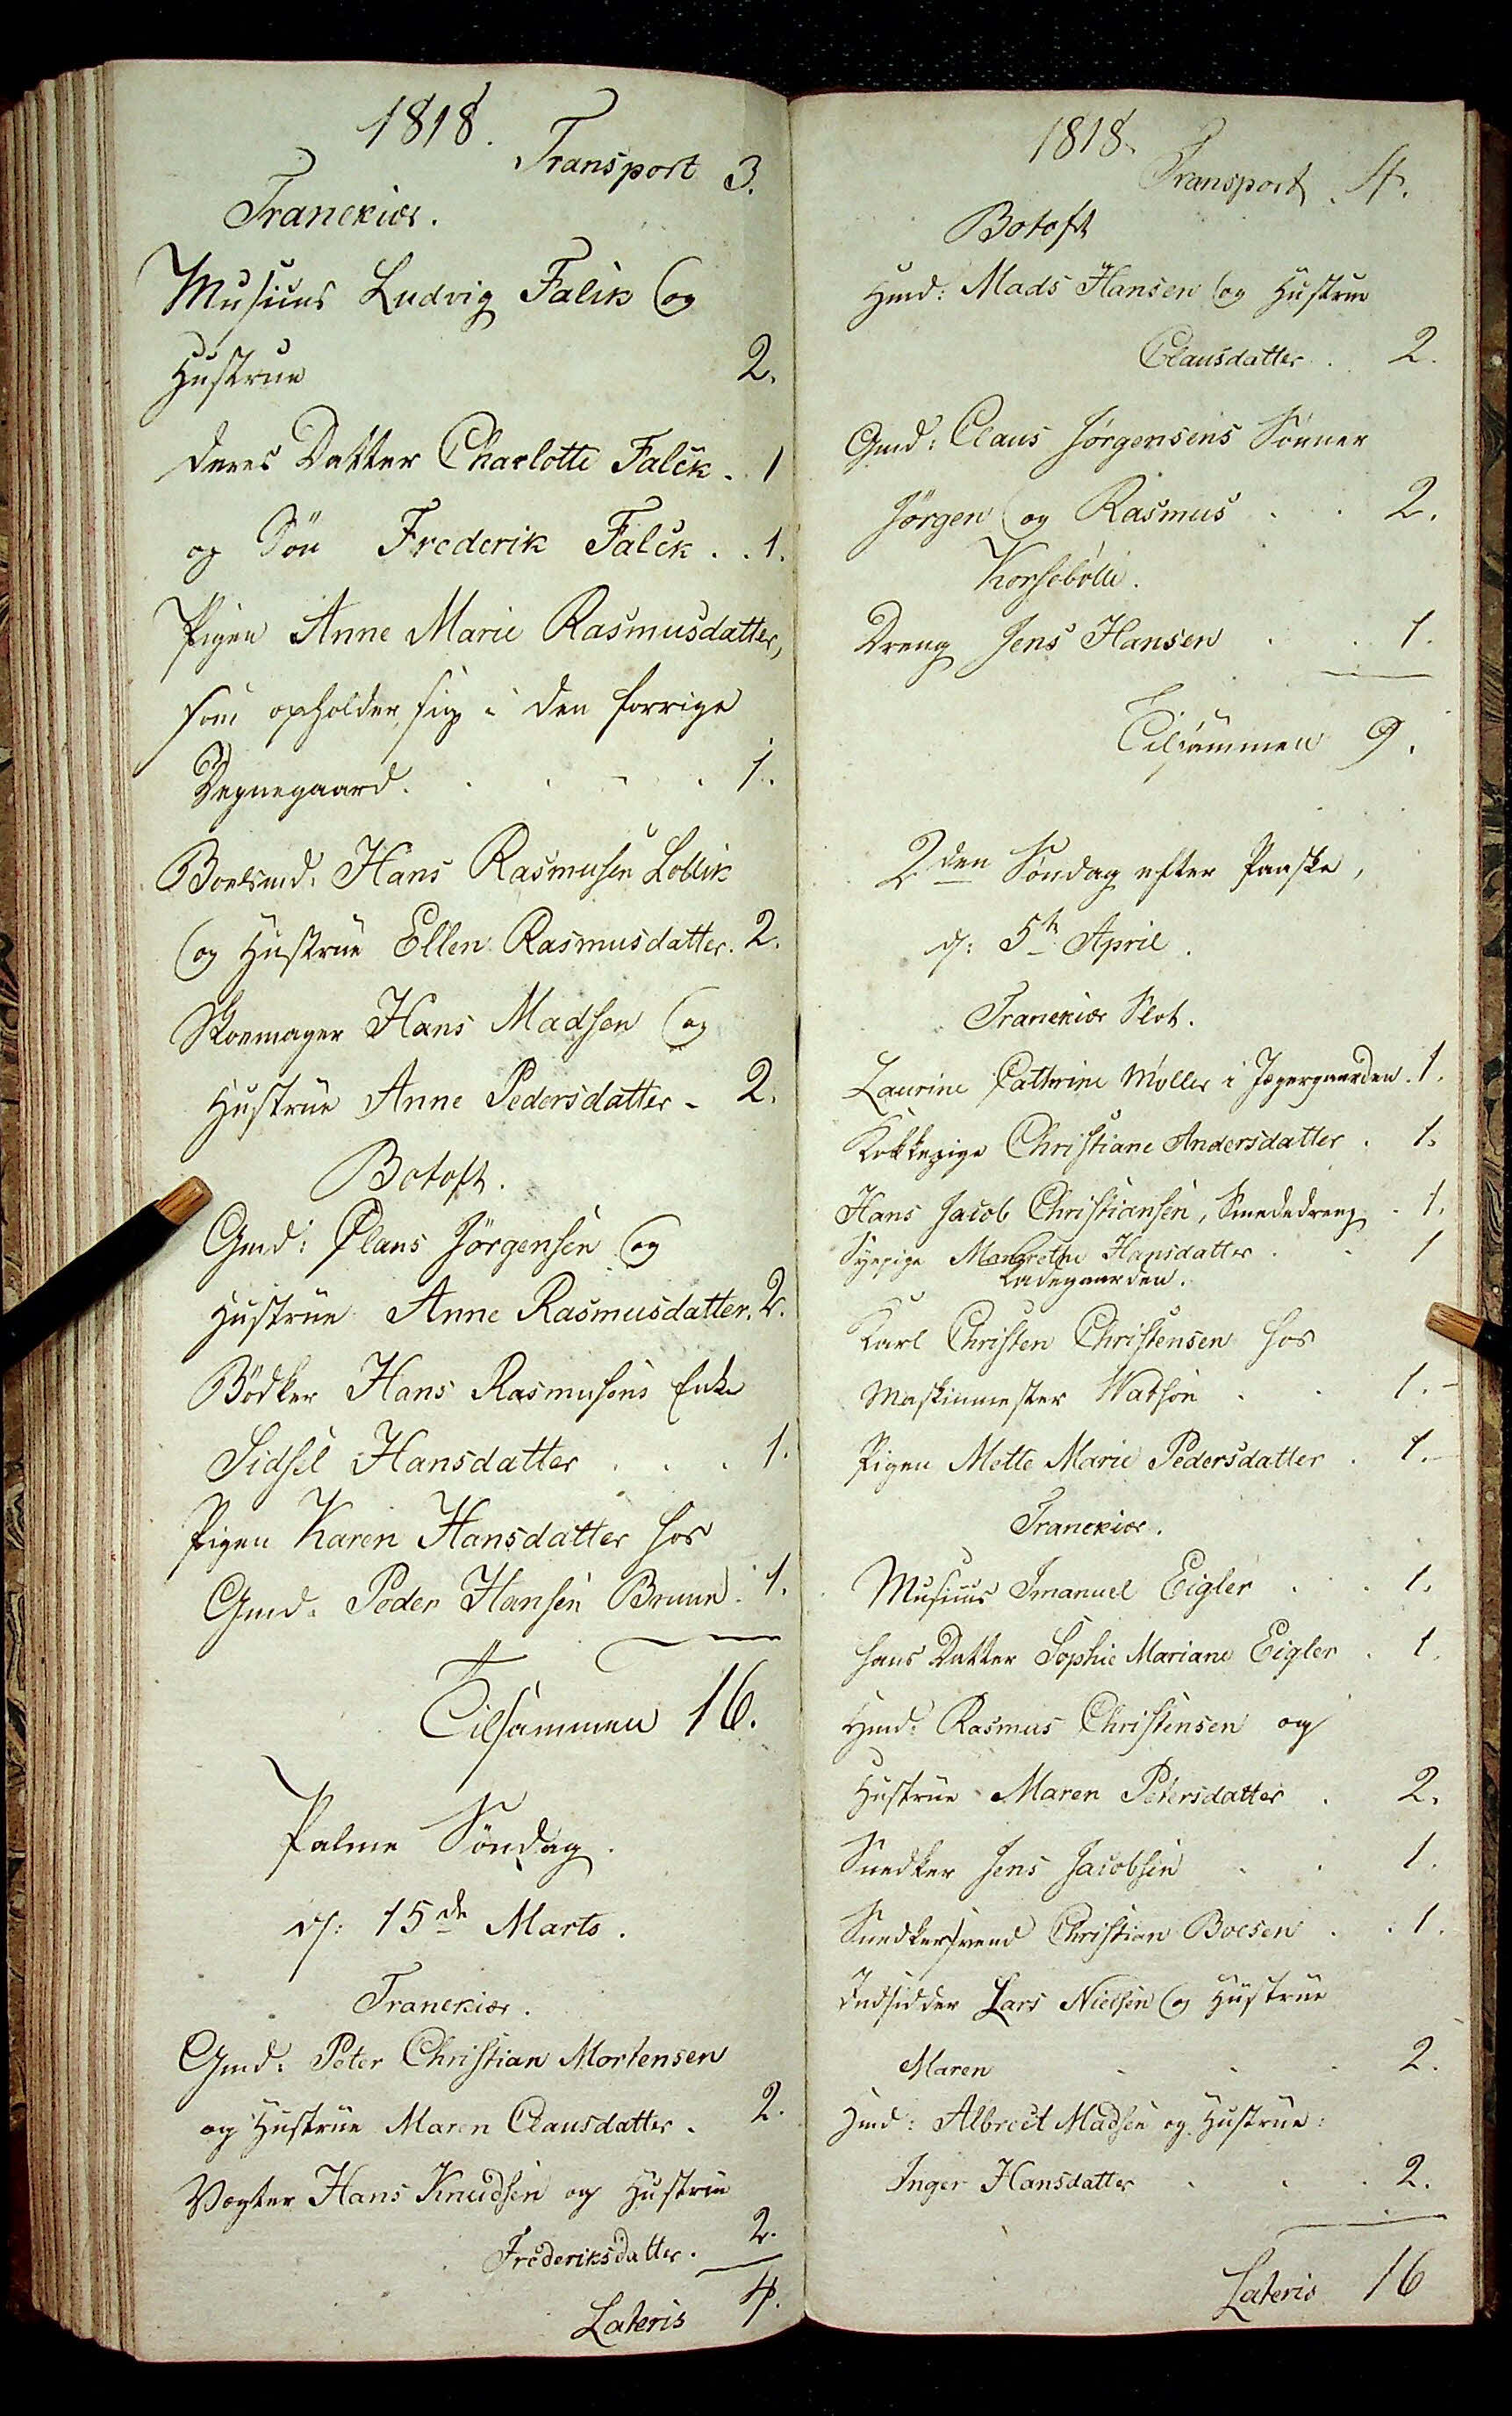1818.
Transport 3.
Tranekiær.
Musicus Ludvig Falck og
Hustrue 2.
deres Datter Charlotte Falck . 1
og Søn Friderik Falck . . 1.
Pigen Anne Marie Rasmusdatter,
som opholder sig i den forrige
Degnegaard . . . . . 1.
Boelsmd: Hans Rasmusen Lollik
og Hustrue Ellen Rasmusdatter . 2.
Skoemager Hans Madsen og
Hustrue Anne Pedersdatter - 2.
Botoft.
Gmd: Claus Jørgensen og
Hustrue Anne Rasmusdatter . 2.
Bødker Hans Rasmusens Enke
Sidsel Hansdatter . . . 1.
Pigen Karen Hansdatter hos
Gmd: Peder Hansen Bruun . 1.
Tilsammen 16.
Palme Søndag
d: 15de Marts.
Tranekiær.
Gmd: Peter Christian Mortensen
og Hustrue Maren Clausdatter . 2.
Vægter Hans Knudsen og Hustrue
Frederiksdatter . 2.
Lateris 4.
1818.
Transport . 4.
Botoft
Hmd: Mads Hansen og Hustrue
Clausdatter . 2.
Gmd: Claus Jørgensøns Sønner
Jørgen og Rasmus . . 2.
Korsebølle.
Dreng Jens Hansen . . 1.
Tilsammen 9.
2den Søndag efter Paaske,
d: 5te April
Tranekiær Slot.
Laurine Cathrine Møller i Jægergaarden . 1.
Kokkepige Christiane Andersdatter . 1.
Hans Jacob Christiansen, Smededreng . 1.
Syepige Margrethe Hansdatter . . 1
Ladegaarden.
Karl Christen Christensen hos
Maskinmester Watson . . 1.
Pigen Mette Marie Pedersdatter . 1.
Tranekiær.
Musicus Imanuel Eigler . . 1.
hans Datter Sophie Mariane Eigler . 1.
Hmd: Rasmus Christensen og
Hustrue Maren Petersdatter . 2.
Snedker Jens Jacobsen . . 1
Snedkersvend Christian Boesen . . 1
Indsidder Lars Nielsen og Hustrue
Maren . . . 2.
Hmd: Albrect Madsen og Hustrue:
Inger Hansdatter . . . 2.
Lateris 16
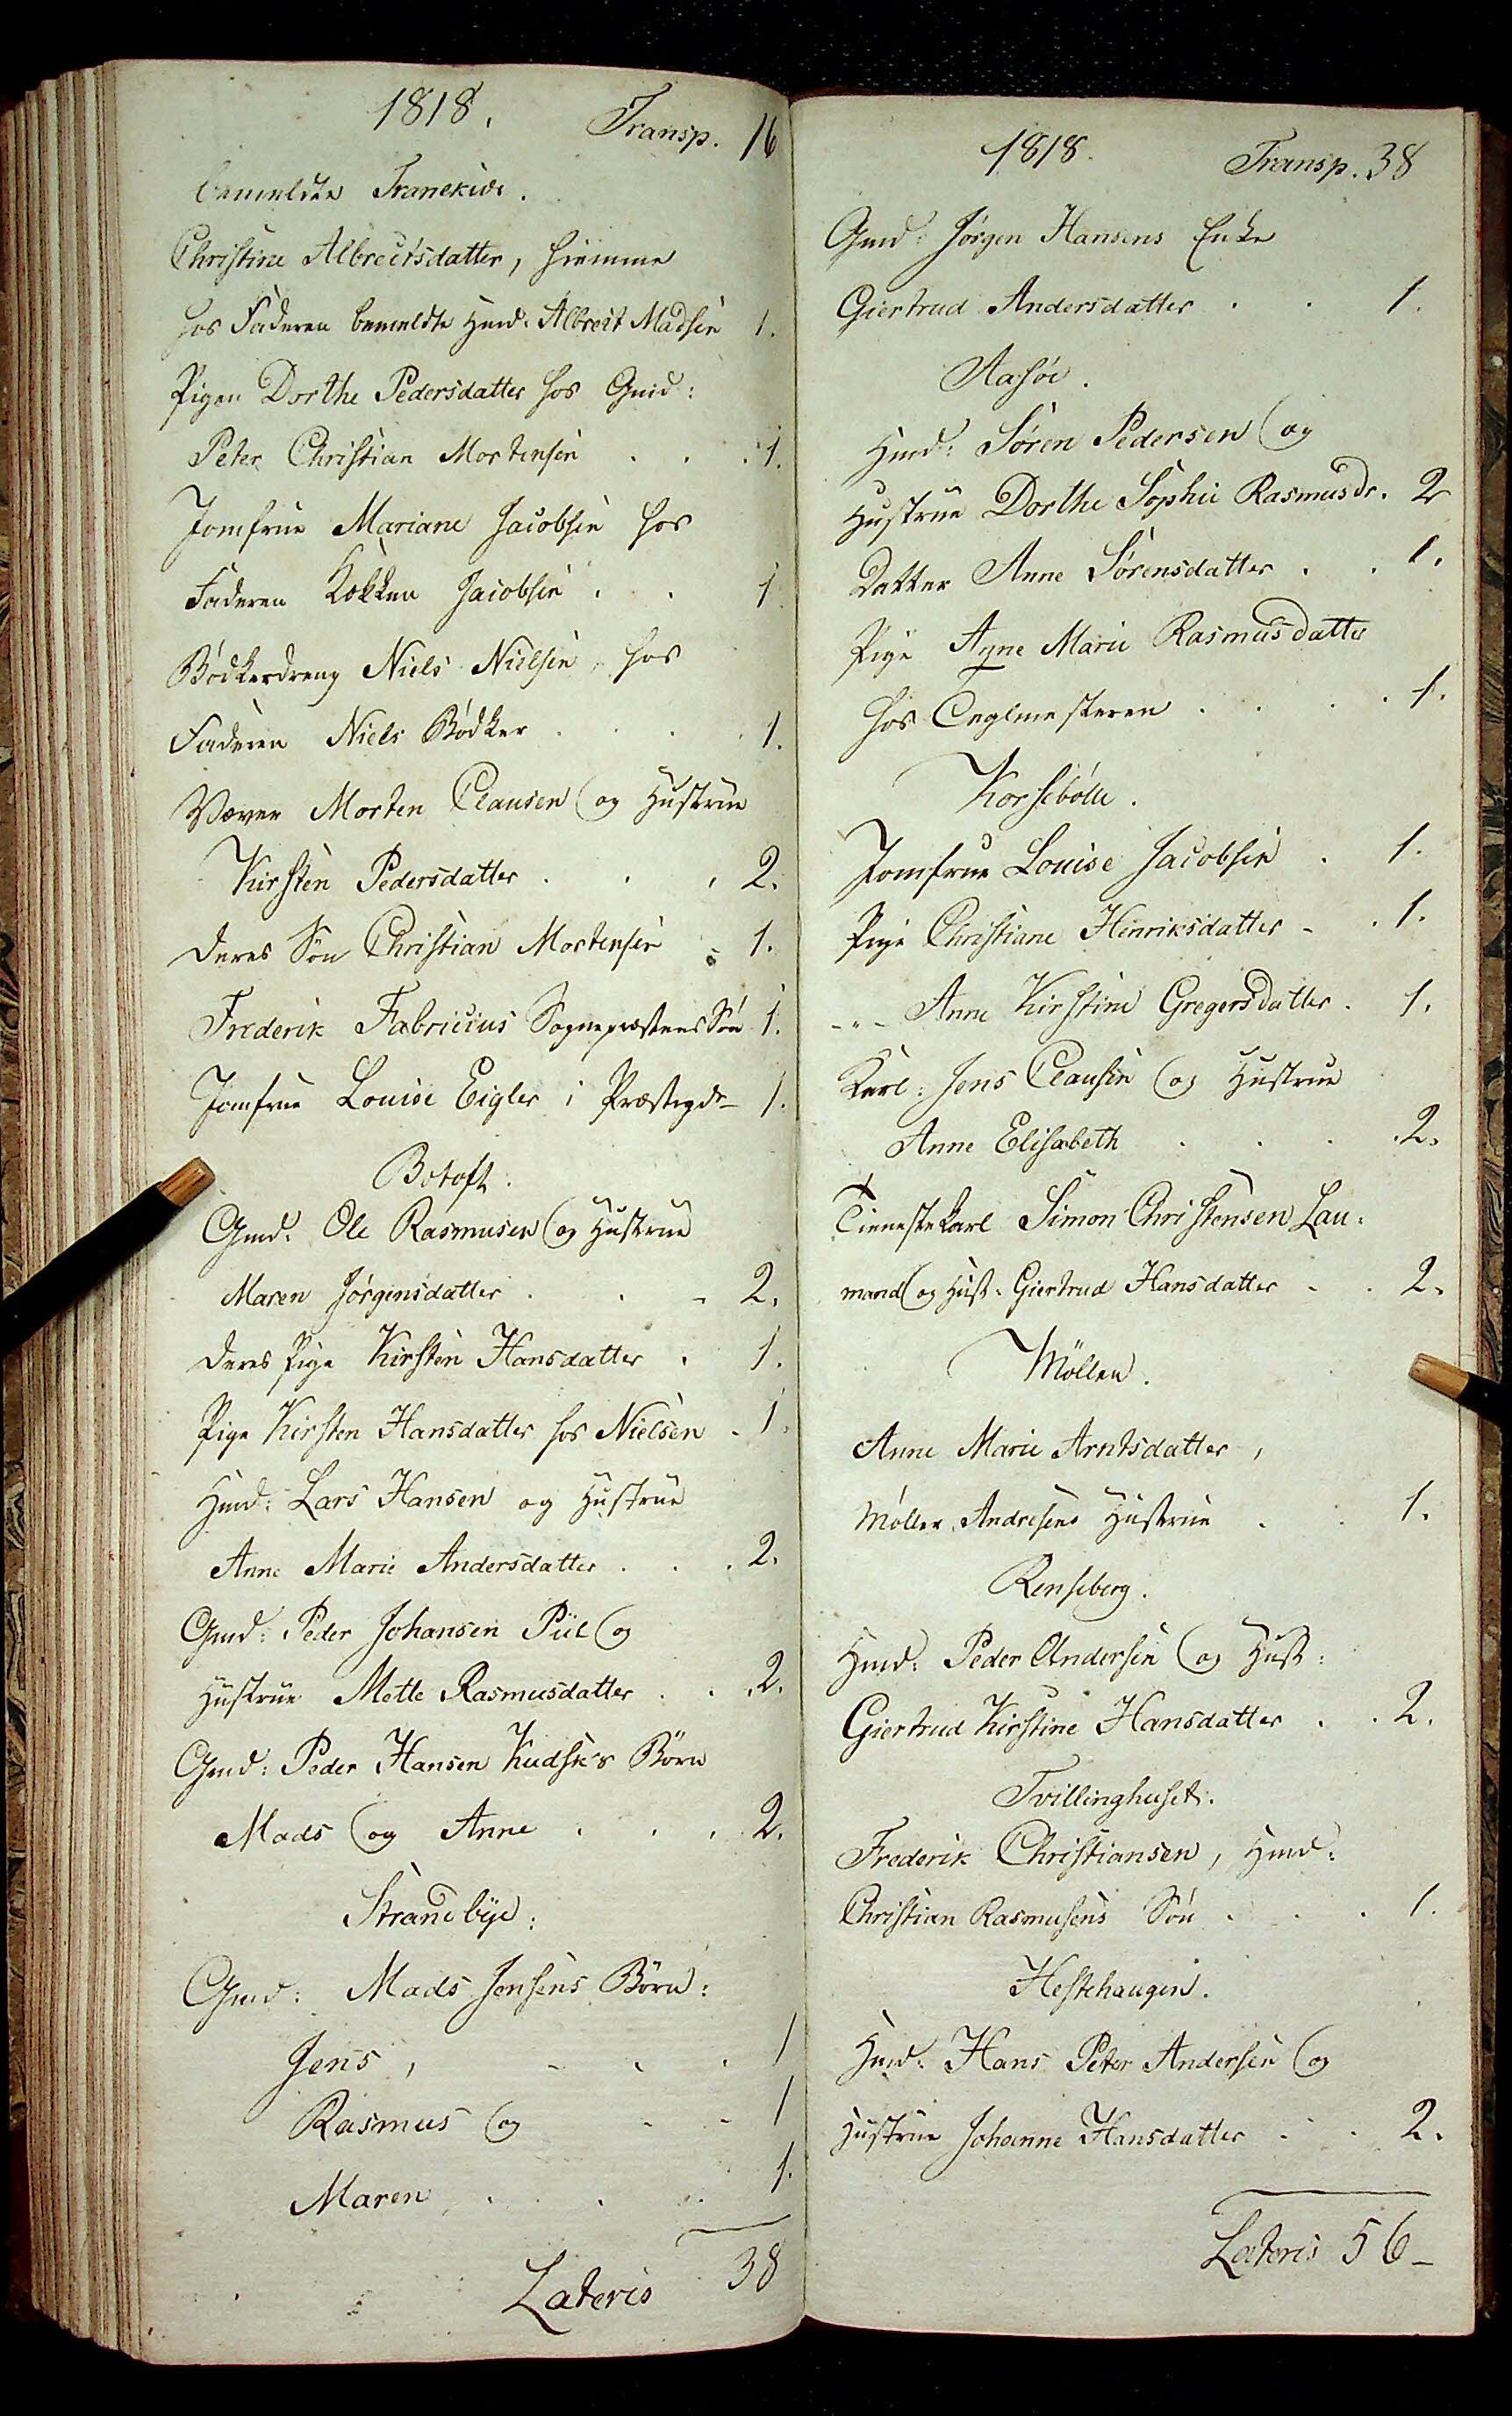1818.
Transp. 16
bemeldte Tranekiær.
Christine Albrectsdatter, hiemme
hos Faderen bemeldte Hmd: Albrect Madsen 1.
Pigen Dorthe Pedersdatter hos Gmd:
Peter Christian Mortensen . . . 1.
Jomfrue Mariane Jacobsen hos
Faderen Kokken Jacobsen . . 1.
Bødkerdreng Niels Nielsen, hos
Faderen Niels Bødker . . . . 1.
Væver Morten Clausen og Hustrue
Kirsten Pedersdatter . . . 2.
deres Søn Christian Mortensen . 1.
Friderik Fabricius Sognepræstens Søn 1.
Jomfrue Louise Eigler i Præstegdr 1.
Botoft
Gmd: Ole Rasmusen og Hustrue
Maren Jørgensdatter . . . 2.
deres Pige Kirsten Hansdatter . 1.
Pige Kirsten Hansdatter hos Nielsen . 1.
Hmd: Lars Hansen og Hustrue
Anne Marie Andersdatter . . . 2.
Gmd: Peder Johansen Pul og
Hustrue Mette Rasmusdatter . . . 2.
Gmd: Peder Hansen Kudsk's Børn
Mads og Anne . . . 2.
Strandbye:
Gmd: Mads Jensens Børn:
Jens . . . . 1
Rasmus og . . 1
Maren . . . . 1.
Lateris 38
1818.
Transp. 38
Gud: Jørgen Hansens Enke
Giertrud Andersdatter . . 1.
Aasøe.
Hmd: Søren Pedersen og
Hustrue Dorthe Sophie Rasmusdr .  2
Datter Anne Sørensdatter . .1.
Pige Anne Marie Rasmusdatter
hos Teglmesteren . . . . 1.
Korsebølle.
Jomfrue Louise Jacobsen . 1.
Pige Christiane Henriksdatter . . 1.
-"- Anne Kirstine Gregersdatter . 1.
Karl: Jens Clausen og Hustrue.
Anne Elisabeth . . . . 2.
Tienestekarl Simon Christensens Lau¬
mand og Hust: Giertrud Hansdatter . . 2.
Møllen.
Anne Marie Arntsdatter
Møller Andersens Hustrue . . 1.
Renseberg.
Hmd: Peder Andersen og Hust:
Giertrud Kirstine Hansdatter . . 2.
Tvillinghuset.
Frederik Christiansen, Hmd:
Christian Rasmusens Søn . . . 1.
Hestehaugen.
Hmd: Hans Peter Andersen og
Hustrue Johanne Hansdatter . . 2.
Lateris 56-